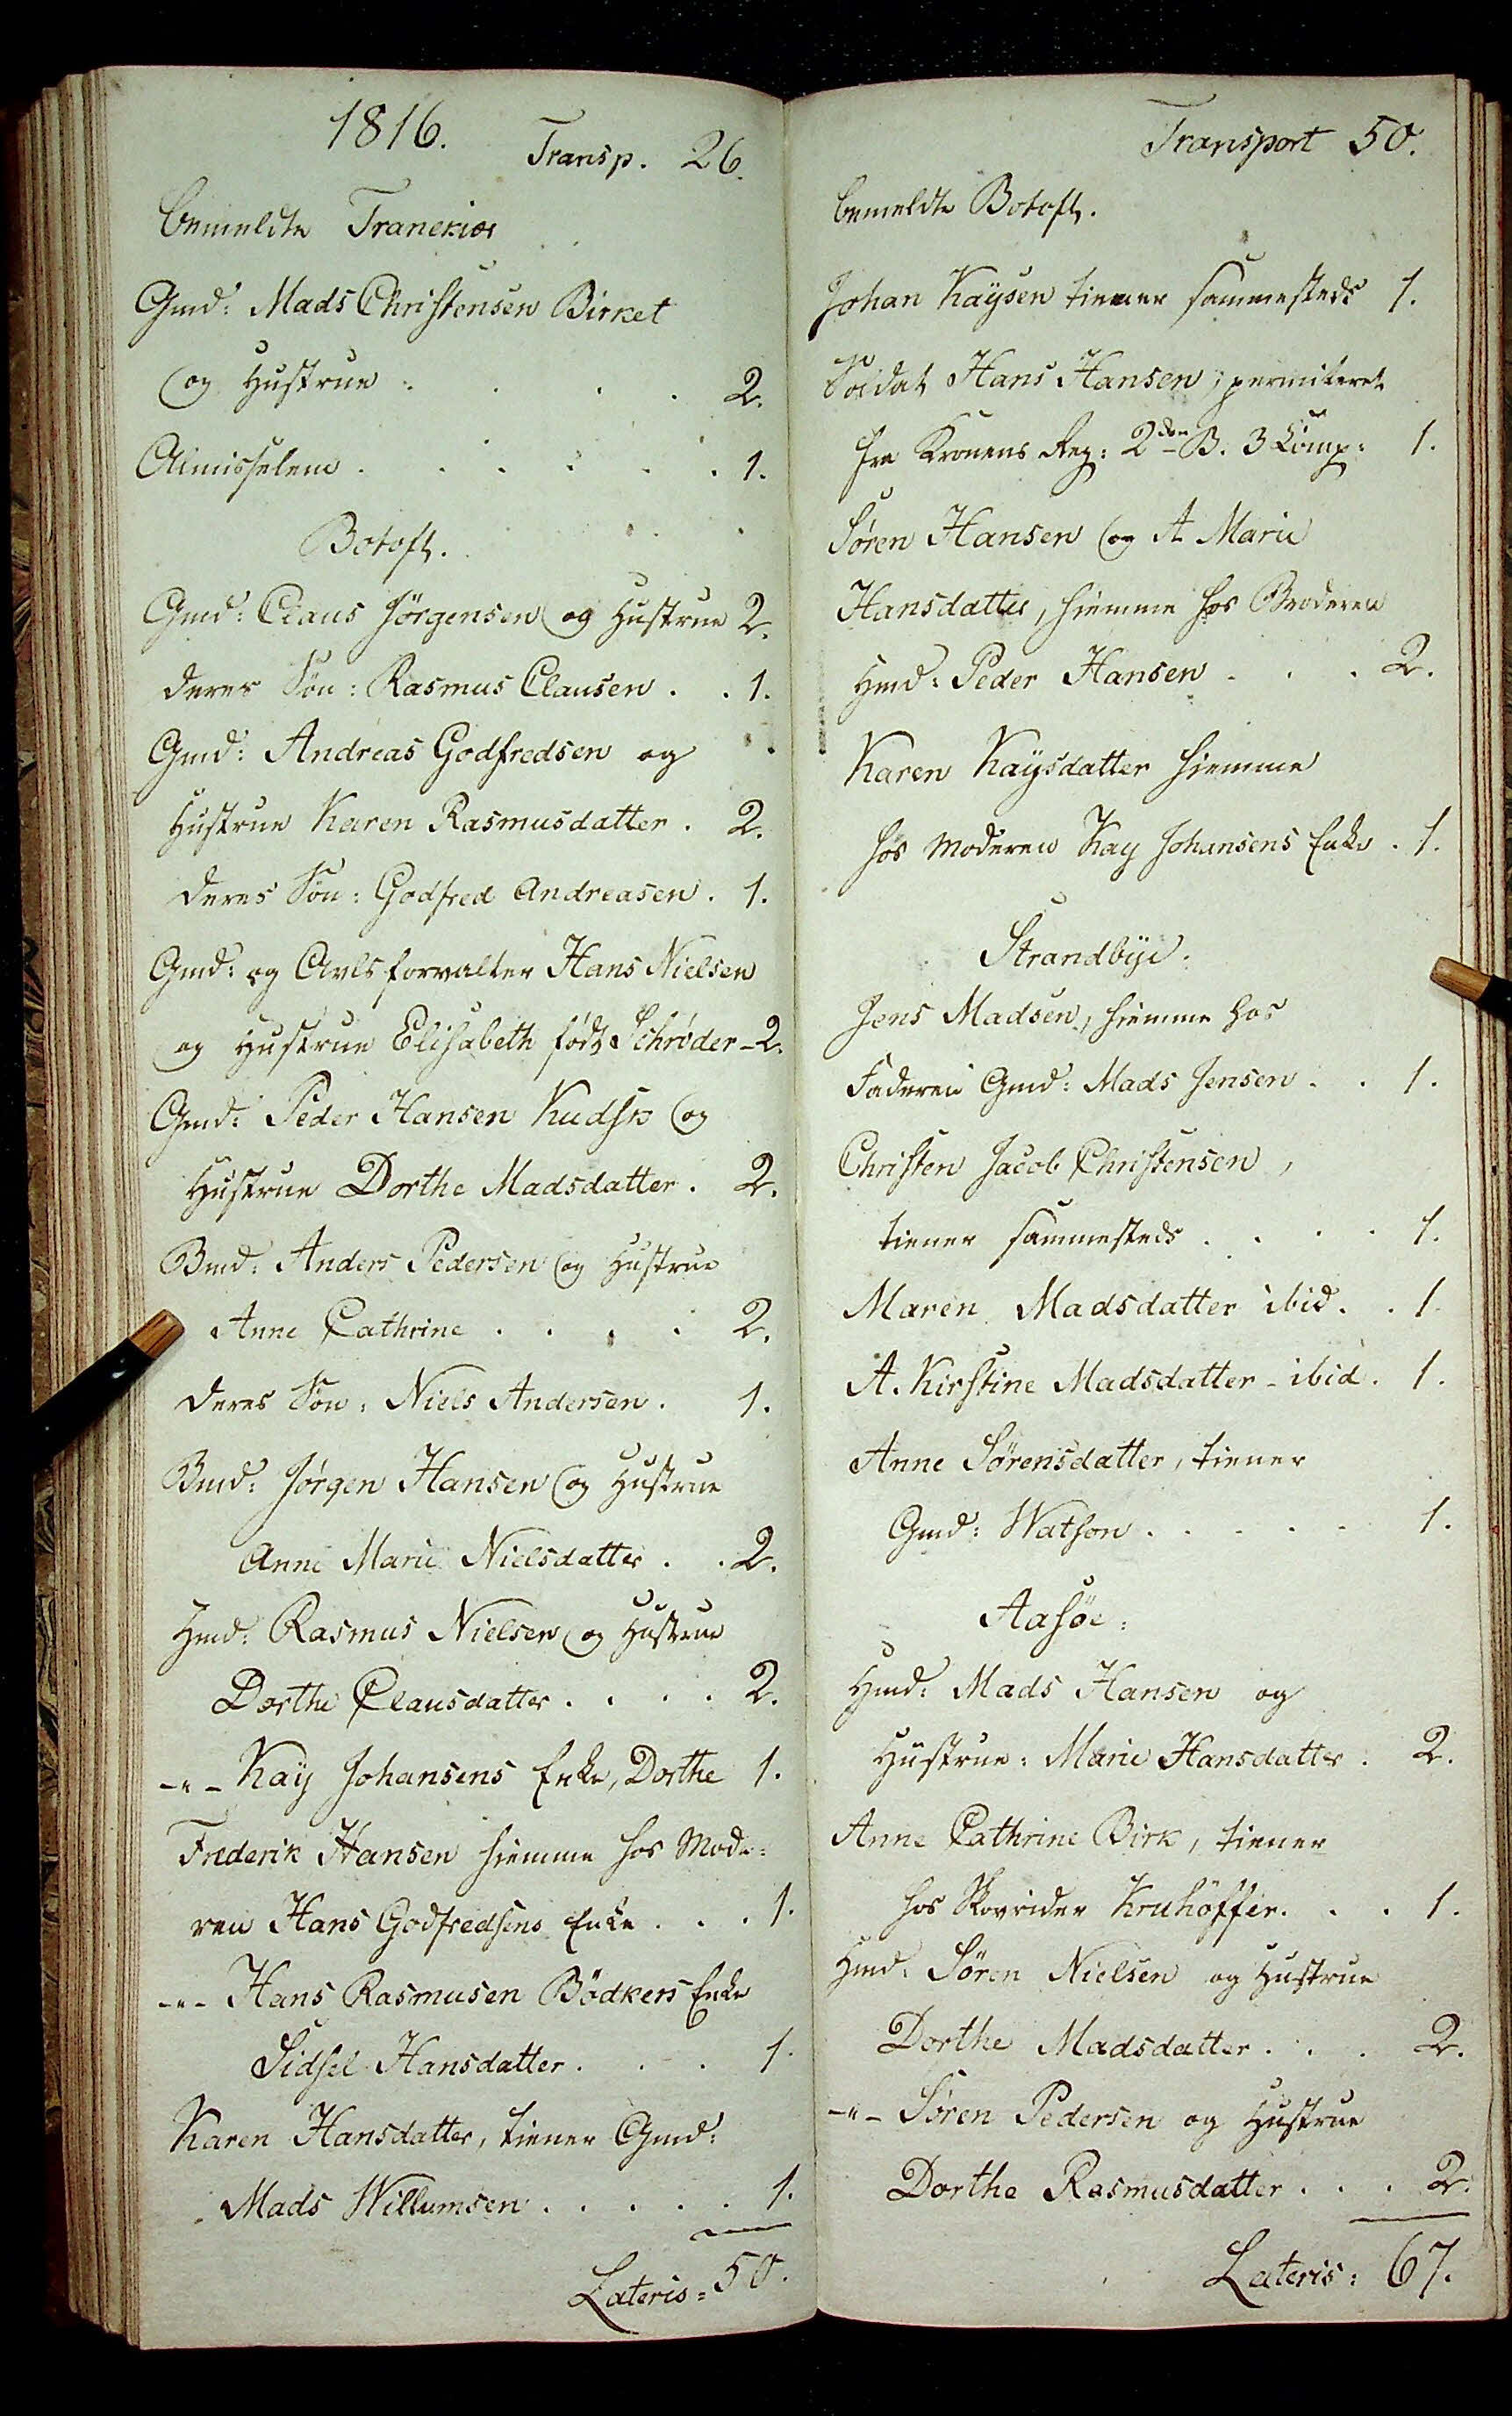1816.
Transp. 26.
bemeldte Tranekiær
Gmd: Mads Christensen Birket
og Hustrue : . . . 2.
Almisselem. . . . . . 1.
Botoft.
Gmd: Claus Jørgensøn og Hustrue 2.
deres Søn: Rasmus Clausen . . 1.
Gmd: Andreas Godfredsen og
Hustrue Karen Rasmusdatter . 2.
deres Søn: Godfred Andreasen . 1.
Gmd: og Avlsforvalter Hans Nielsen
og Hustrue Elisabeth født Schrøder - 2.
Gmd: Peder Hansen Kudsk og
Hustrue Dorthe Madsdatter . 2.
Bmd: Anders Pedersen og Hustrue
Anne Cathrine . . . . 2.
deres Søn: Niels Andersen . 1.
Bmd: Jørgen Hansen og Hustrue
Anne Marie Nielsdatter . . 2.
Hmd: Rasmus Nielsen og Hustrue
Dorthe Clausdatter . . . . 2.
-"- Kay Johansens Enke, Dorthe 1.
Frederik Hansen hiemme hos Mode¬
ren Hans Godredsens Enke . . . 1
-"- Hans Rasmusen Bødkers Enke
Sidsel Hansdatter . . . 1
Karen Hansdatter, tiener Gmd:
Mads Willumsen . . . . . 1
Lateris = 50.
Transport 50.
bemeldte Botoft.
Johan Kaysen tiener sammesteds 1.
Soldat Hans Hansen; permiteret
fra Kronens Reg: 2den B. 3. Komp : 1
Søren Hansen og A. Marie
Hansdatter, hiemme hos Broderen
Hmd: Peder Hansen . . . 2.
Karen Kaysdatter hiemme
hos Moderen Kay Johansens Enke . 1.
Strandbye.
Jens Madsen, hiemme hos
Faderen Gmd: Mads Jensen . . 1.
Christen Jacob Christensen,
tiener sammesteds . . . . 1.
Maren Madsdatter ibid . . 1
A. Kirstine Madsdatter - ibid . 1.
Anne Sørensdatter, tiener
Gmd: Watson . . . . . 1.
Aasøe:
Hmd: Mads Hansen og
Hustrue: Marie Hansdatter . 2.
Anne Cathrine Birk, tiener
hos Skovrider Kruhøffer . . . 1.
Hmd: Søren Nielsen og Hustrue
Dorthe Madsdatter . . . 2.
-"- Søren Pedersen og Hustrue
Dorthe Rasmusdatter . . . 2
Lateris: 67.
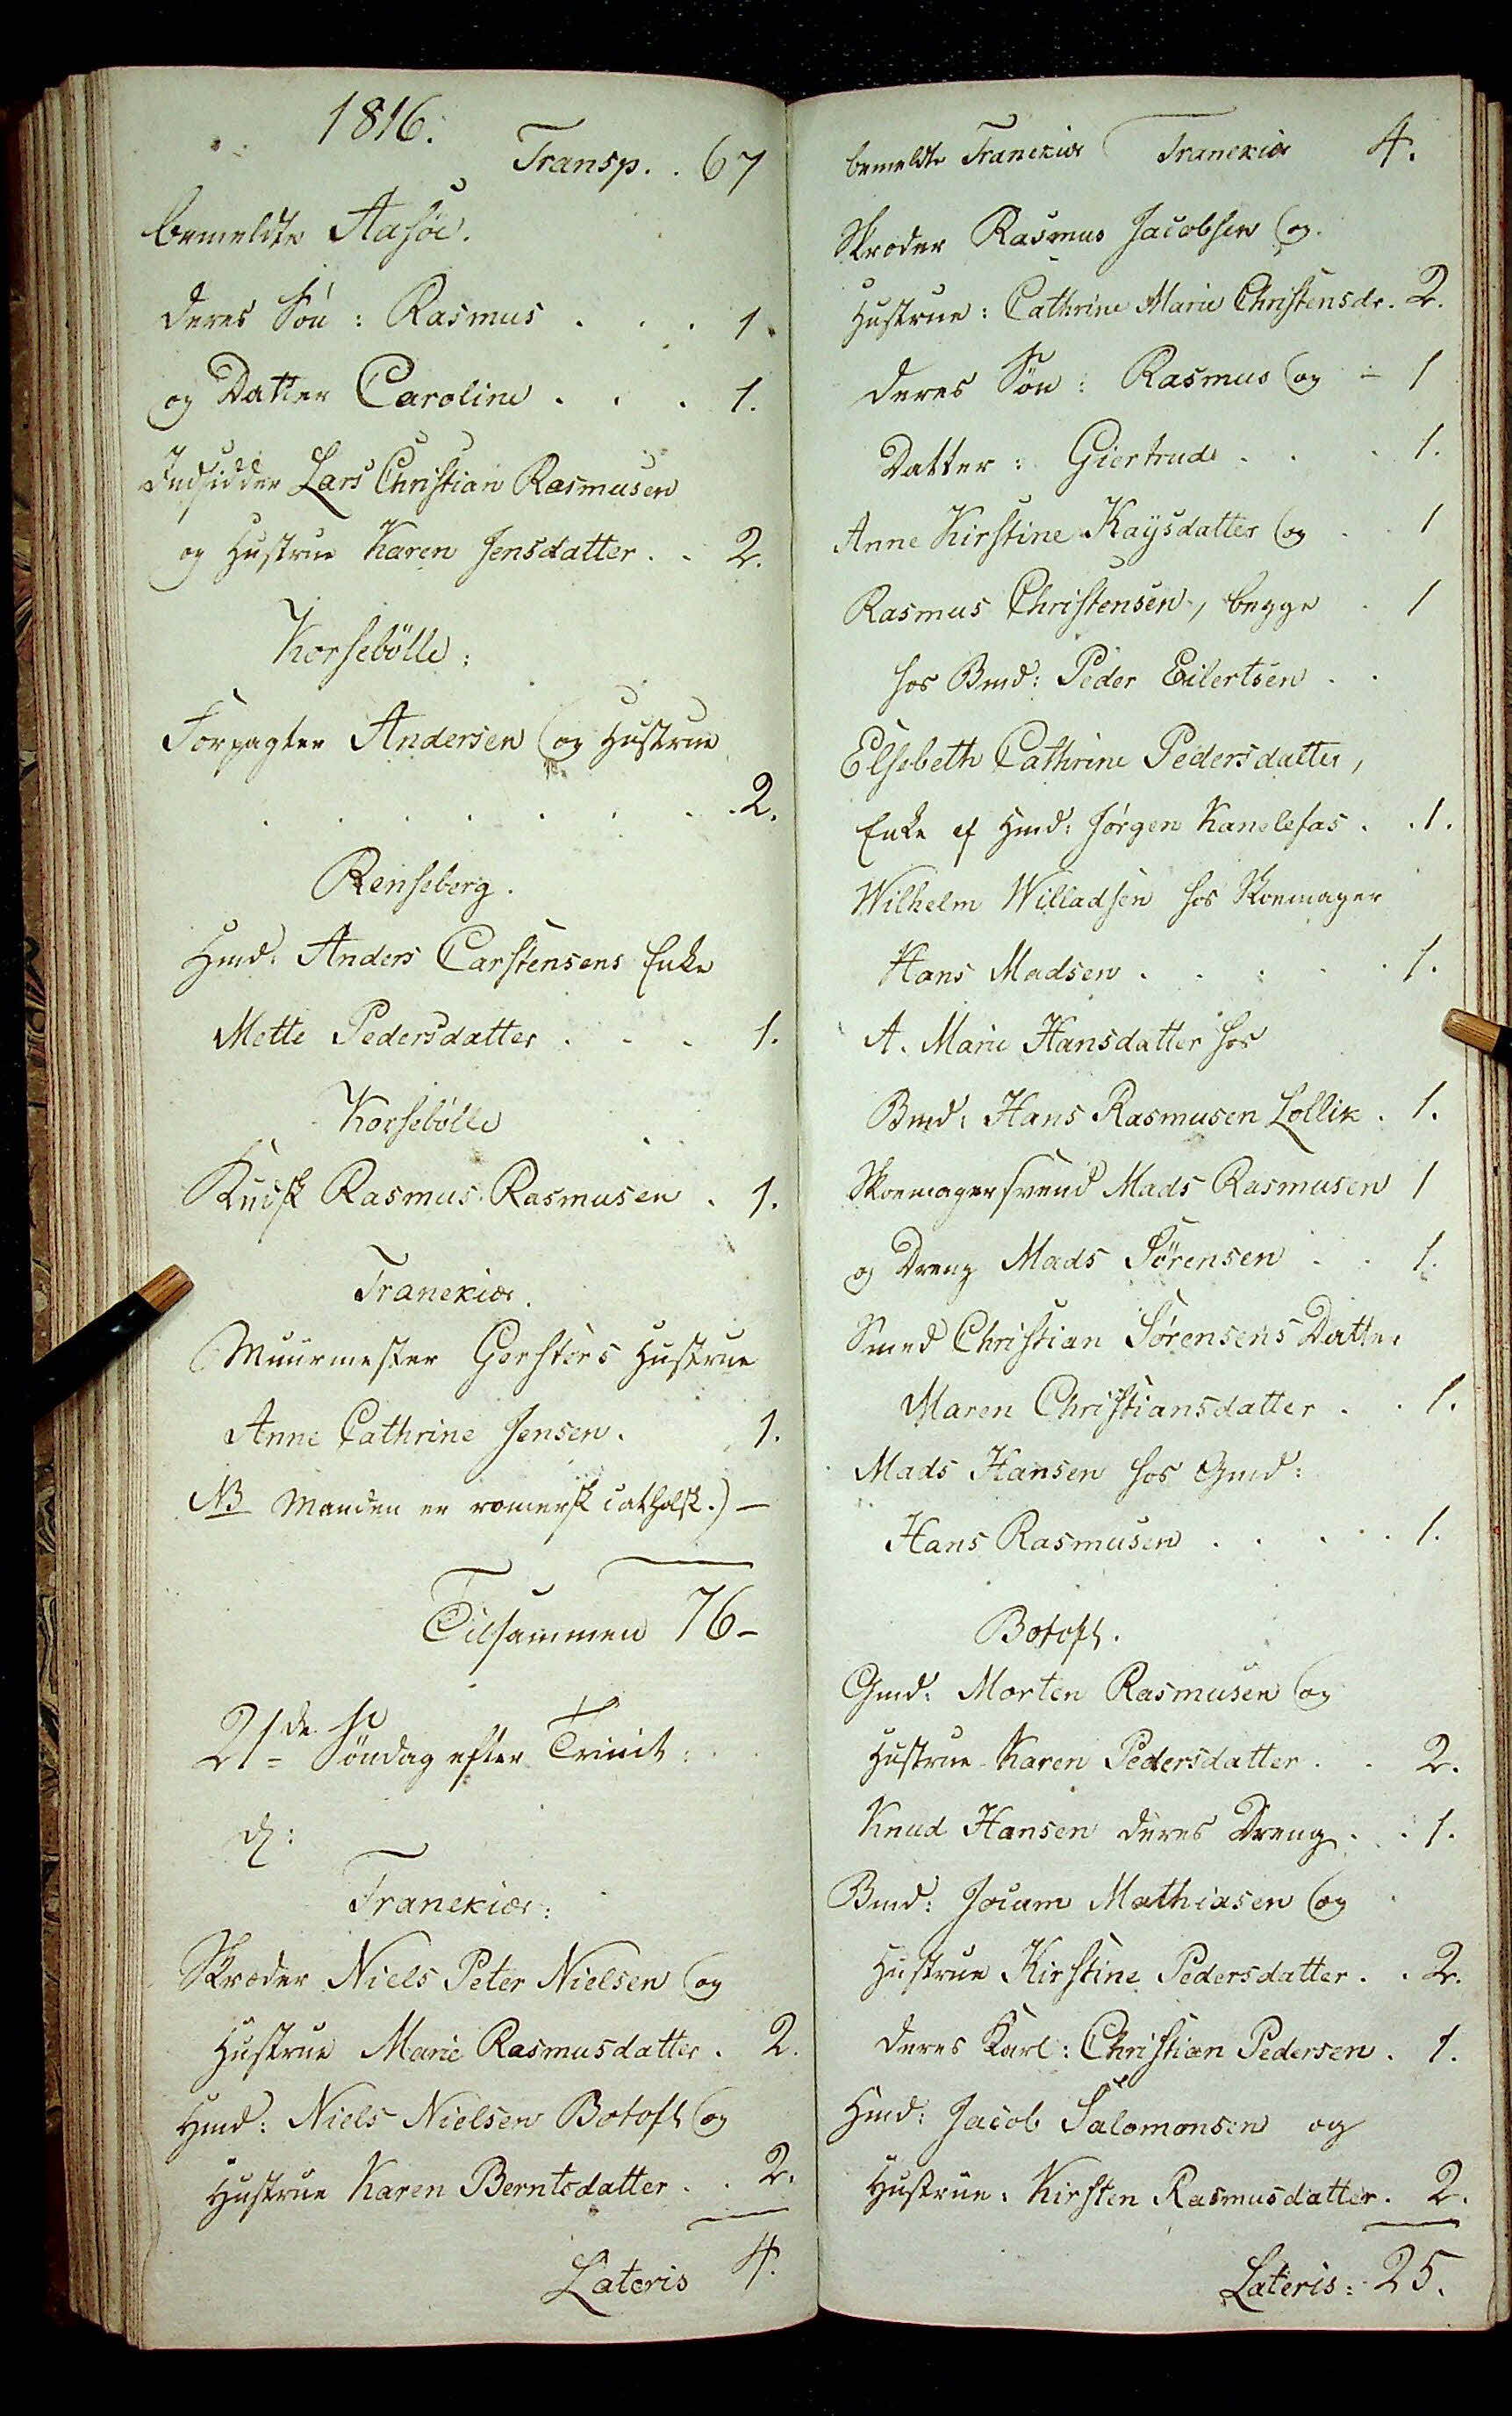1816.
Transp . . 67
bemmeldte Aasøe.
deres Søn: Rasmus . . . 1.
og Datter Carolin . . . 1.
Indsidder Lars Christian Rasmusen
og Hustrue Karen Jensdatter . . 2.
Korsebølle:
Forpagter Andersen og Hustrue
. 2.
Renseberg.
Hmd. Anders Carstensens Enke
Mette Pedersdatter . . . 1.
Korsebølle
Kudsk Rasmus Rasmusen . 1.
Tranekiær.
Muurmester Gersters Hustrue
Anne Cathrine Jensen . 1.
NB Manden er romersk catholsk.) -
Tilsammen 76-
21de Søndag efter Trinit:
Tranekiær:
Skræder Niels Peter Nielsen og
Hustrue Marie Rasmusdatter . 2.
Hmd: Niels Nielsen Botoft og
Hustrue Karen Berntsdatter . . 2.
Lateris 4.
bemeldte Tranekiær Tranekiær 4.
Skræder Rasmus Jacobsen og.
Hustrue: Cathrine Marie Christensdr . 2.
deres Søn: Rasmus og - 1
Datter: Giertrud . . . 1.
Anne Kirstine Kaysdatter og . . 1
Rasmus Christensen, begge . 1
hos Bmd: Peder Eilertsen
Elsebeth Cathrine Pedersdatter,
Enke ef Hmd: Jørgen Kanelefas . . 1.
Wilhelm Willadsen hos Skoemager
Hans Madsen . . . . . 1.
A. Marie Hansdatter hos
Bmd: Hans Rasmusen Lollik . 1.
Skoemagersvend Mads Rasmusen 1
og Dreng Mads Sørensen . . 1.
Smed Christian Sørensens Datter:
Maren Christiansdatter . . 1.
Mads Hansen hos Gmd:
Hans Rasmusen . . . . . 1.
Botoft.
Gmd: Morten Rasmusen og
Hustrue Karen Pedersdatter . . 2.
Knud Hansen deres Dreng . . 1.
Bmd: Jocum Mathiasen og
Hustrue Kirstine Pedersdatter . . 2.
deres Karl: Christian Pedersen . 1.
Hmd: Jacob Salomonsen og
Hustrue: Kirsten Rasmusdatter . 2
Lateris: 25.